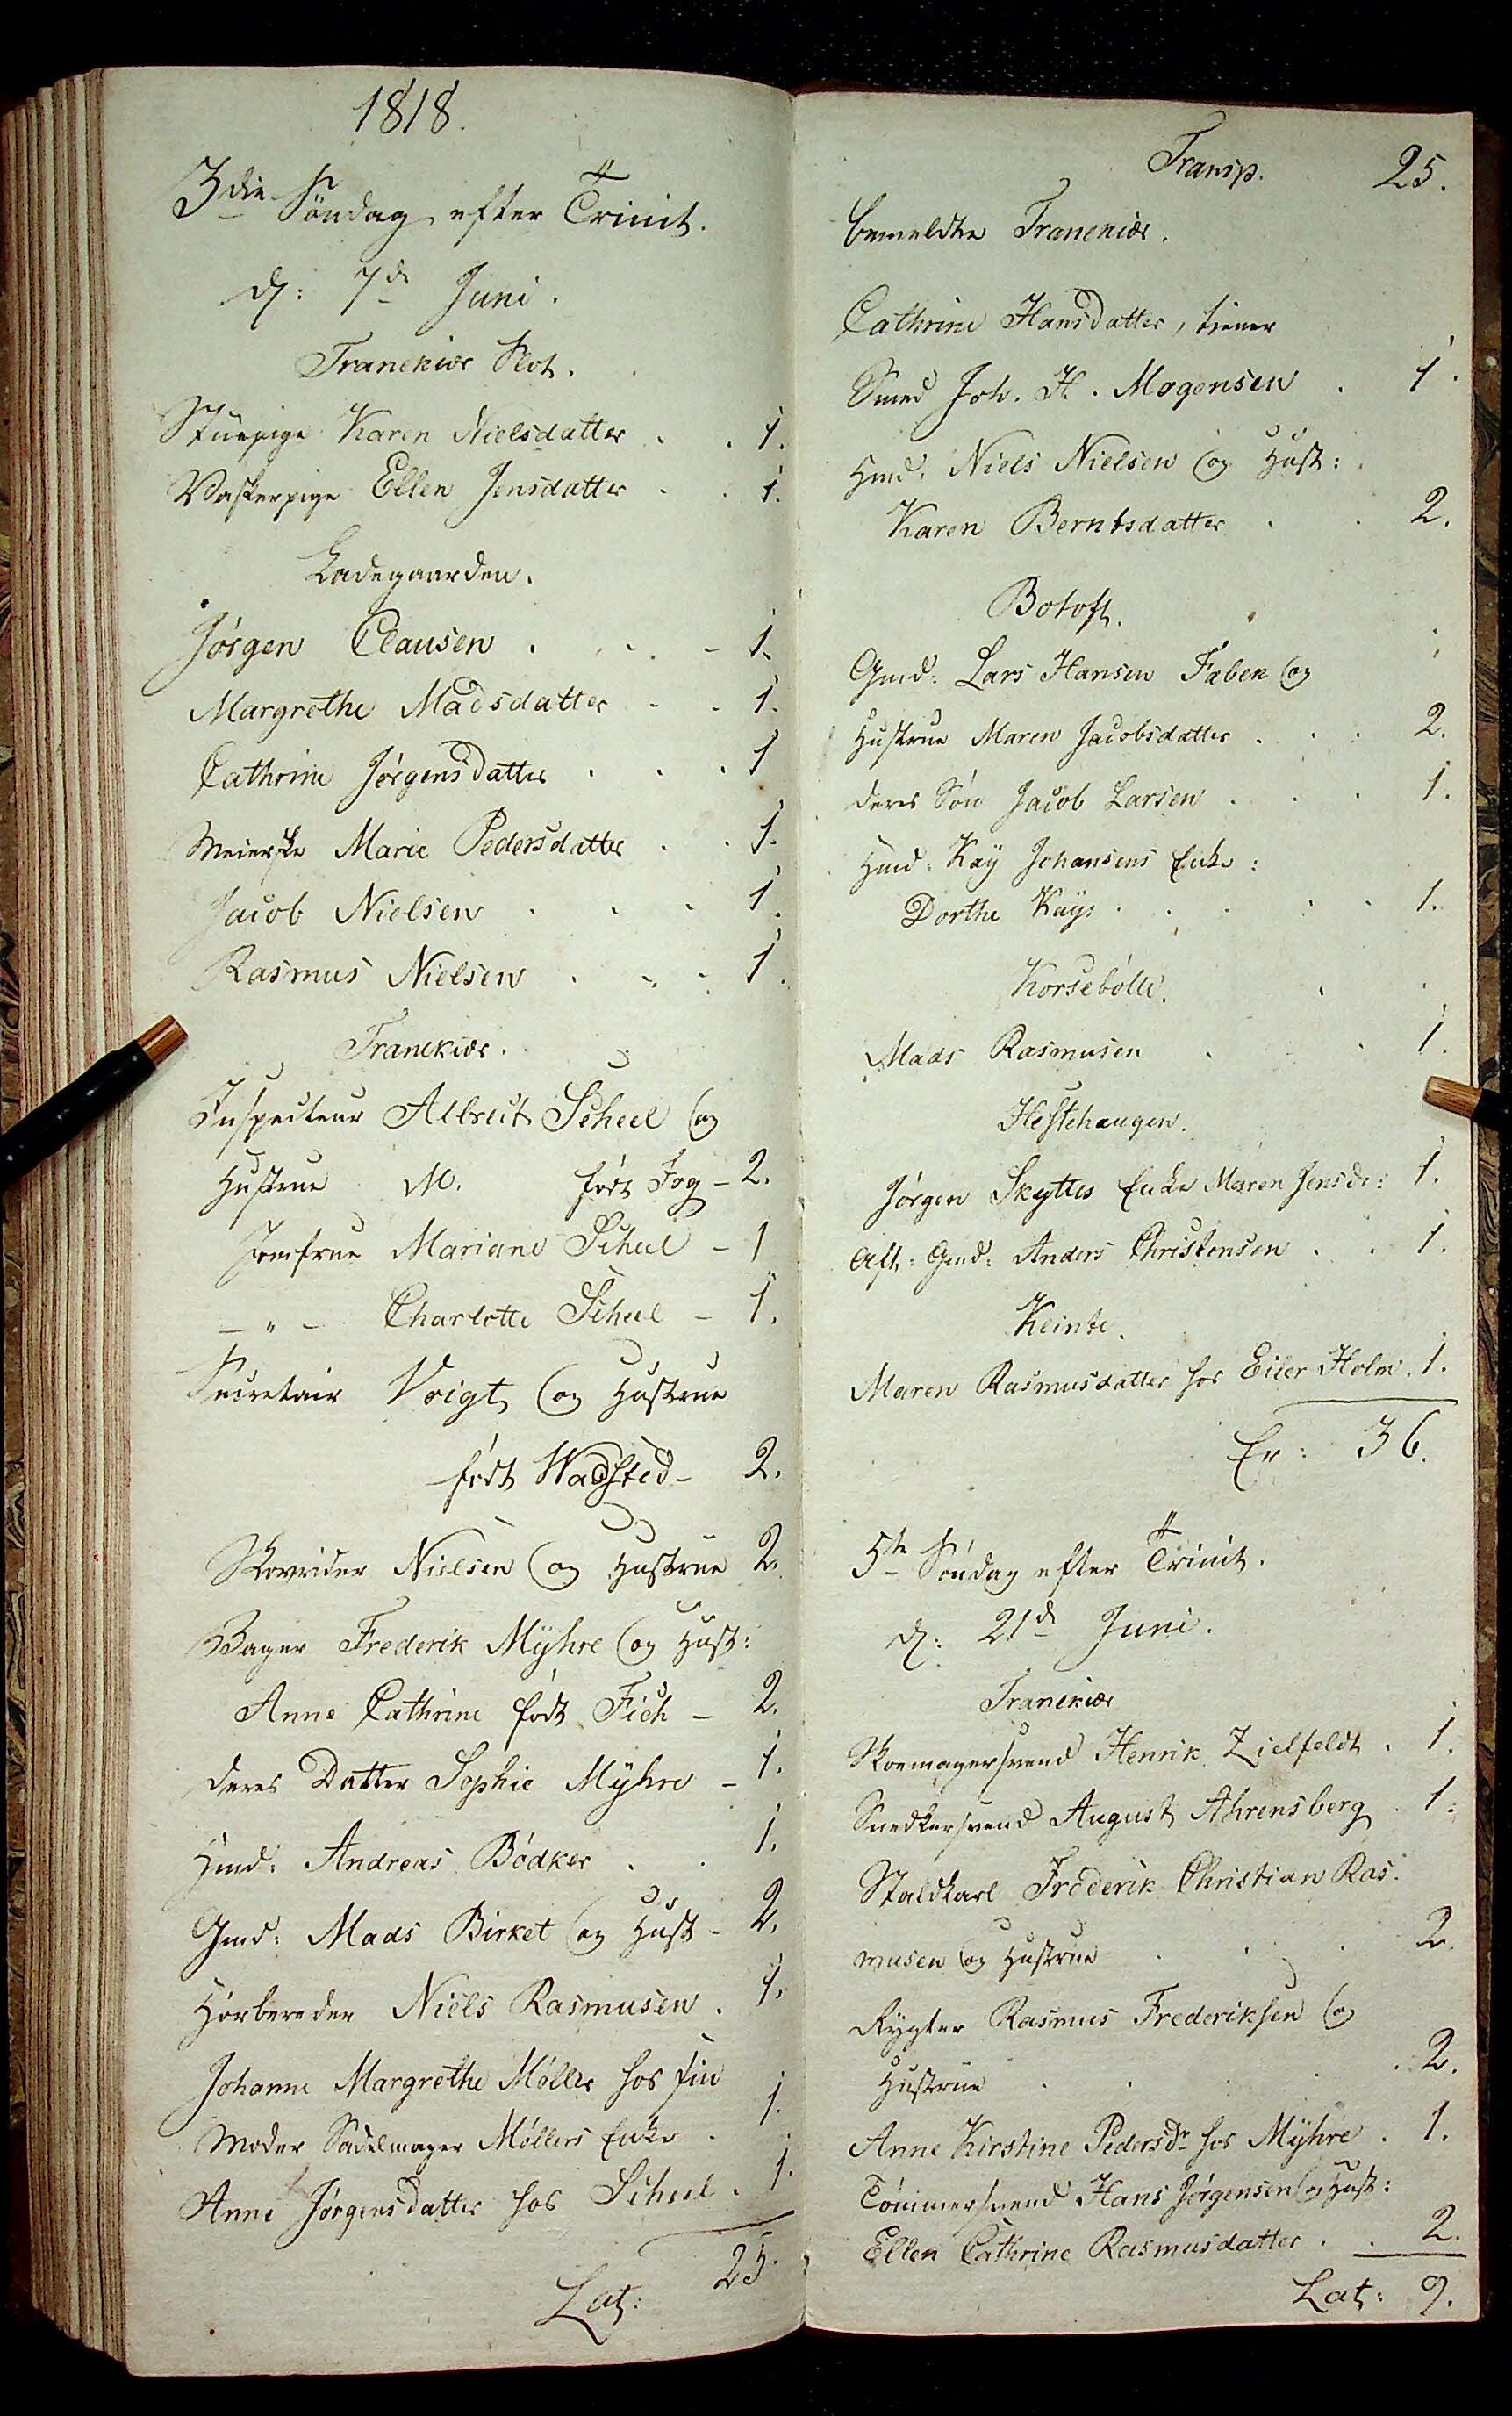1818.
3die Søndag efter Trinit.
d: 7de Juni.
Tranekiær Slot.
Stuepige Karen Nielsdatter . .1.
Vaskerpige Ellen Jensdatter . . 1.
Ladegaarden.
Jørgen Clausen . . . - 1.
Margrethe Madsdatter  . . 1.
Cathrine Jørgensdatter . . . 1.
Meierske Marie Pedersdatter . . 1.
Jacob Nielsen . . . 1.
Rasmus Nielsen . . . 1.
Tranekiær.
Inspecteur Albrect Scheel og
Hustrue M. født Fog - 2.
Jomfrue Mariane Scheel - 1
-"- Charlotte Scheel - 1.
Secretair Voigt og Hustrue
født Wadsted - 2.
Skovrider Nielsen og Hustrue 2.
Bager Frederik Myhre og Hust:
Anne Cathrine født Fich - 2.
deres Datter Sophie Myhre - 1.
Hmd: Andreas Bødker . . 1.
Gmd: Mads Birket og Hust - 2.
Hørbereder Niels Rasmusen . 1.
Johanne Margrethe Møller hos sin
Moder Sadelmager Møllers Enke . 1.
Anne Jørgensdatter hos Scheel . 1.
Lat: 25.
Transp. 25.
bemeldte Tranekiær.
Cathrine Hansdatter, tiener
Smed Joh. H. Mogensen . 1.
Hmd: Niels Nielsen og Hust:
Karen Berntsdatter . . 2.
Botoft.
Gmd: Lars Hansen Fæbek og
Hustrue Maren Jacobsdatter . . . 2.
deres Søn Jacob Larsen . . . 1.
Hmd: Kay Johansens Enke:
Dorthe Kays . . . . . 1.
Korsebølle.
Mads Rasmusen . . 1.
Hestehaugen.
Jørgen Skyttes Enke Maren Jensdr : 1.
Aft: Gmd: Anders Christensen . . 1.
Klinte.
Maren Rasmusdatter hos Eiler Holm . 1.
Er: 36.
5te Søndag efter Trinit.
d:
21de Juni.
Tranekiær
Skomagersvend Henric Zielfeldt . 1.
Snedkersvend August Ahrensberg . 1.
Staldkarl Friderik Christian Ras¬
musen og Hustrue . . . 2.
Rygter Rasmus Frederiksen og
Hustrue . . . . . 2.
Anne Kirstine Pedersdr hos Myhre . 1.
Tømmersvend Hans Jørgensen og Hust:
Ellen Cathrine Rasmusdatter . . 2.
Lat: 9.
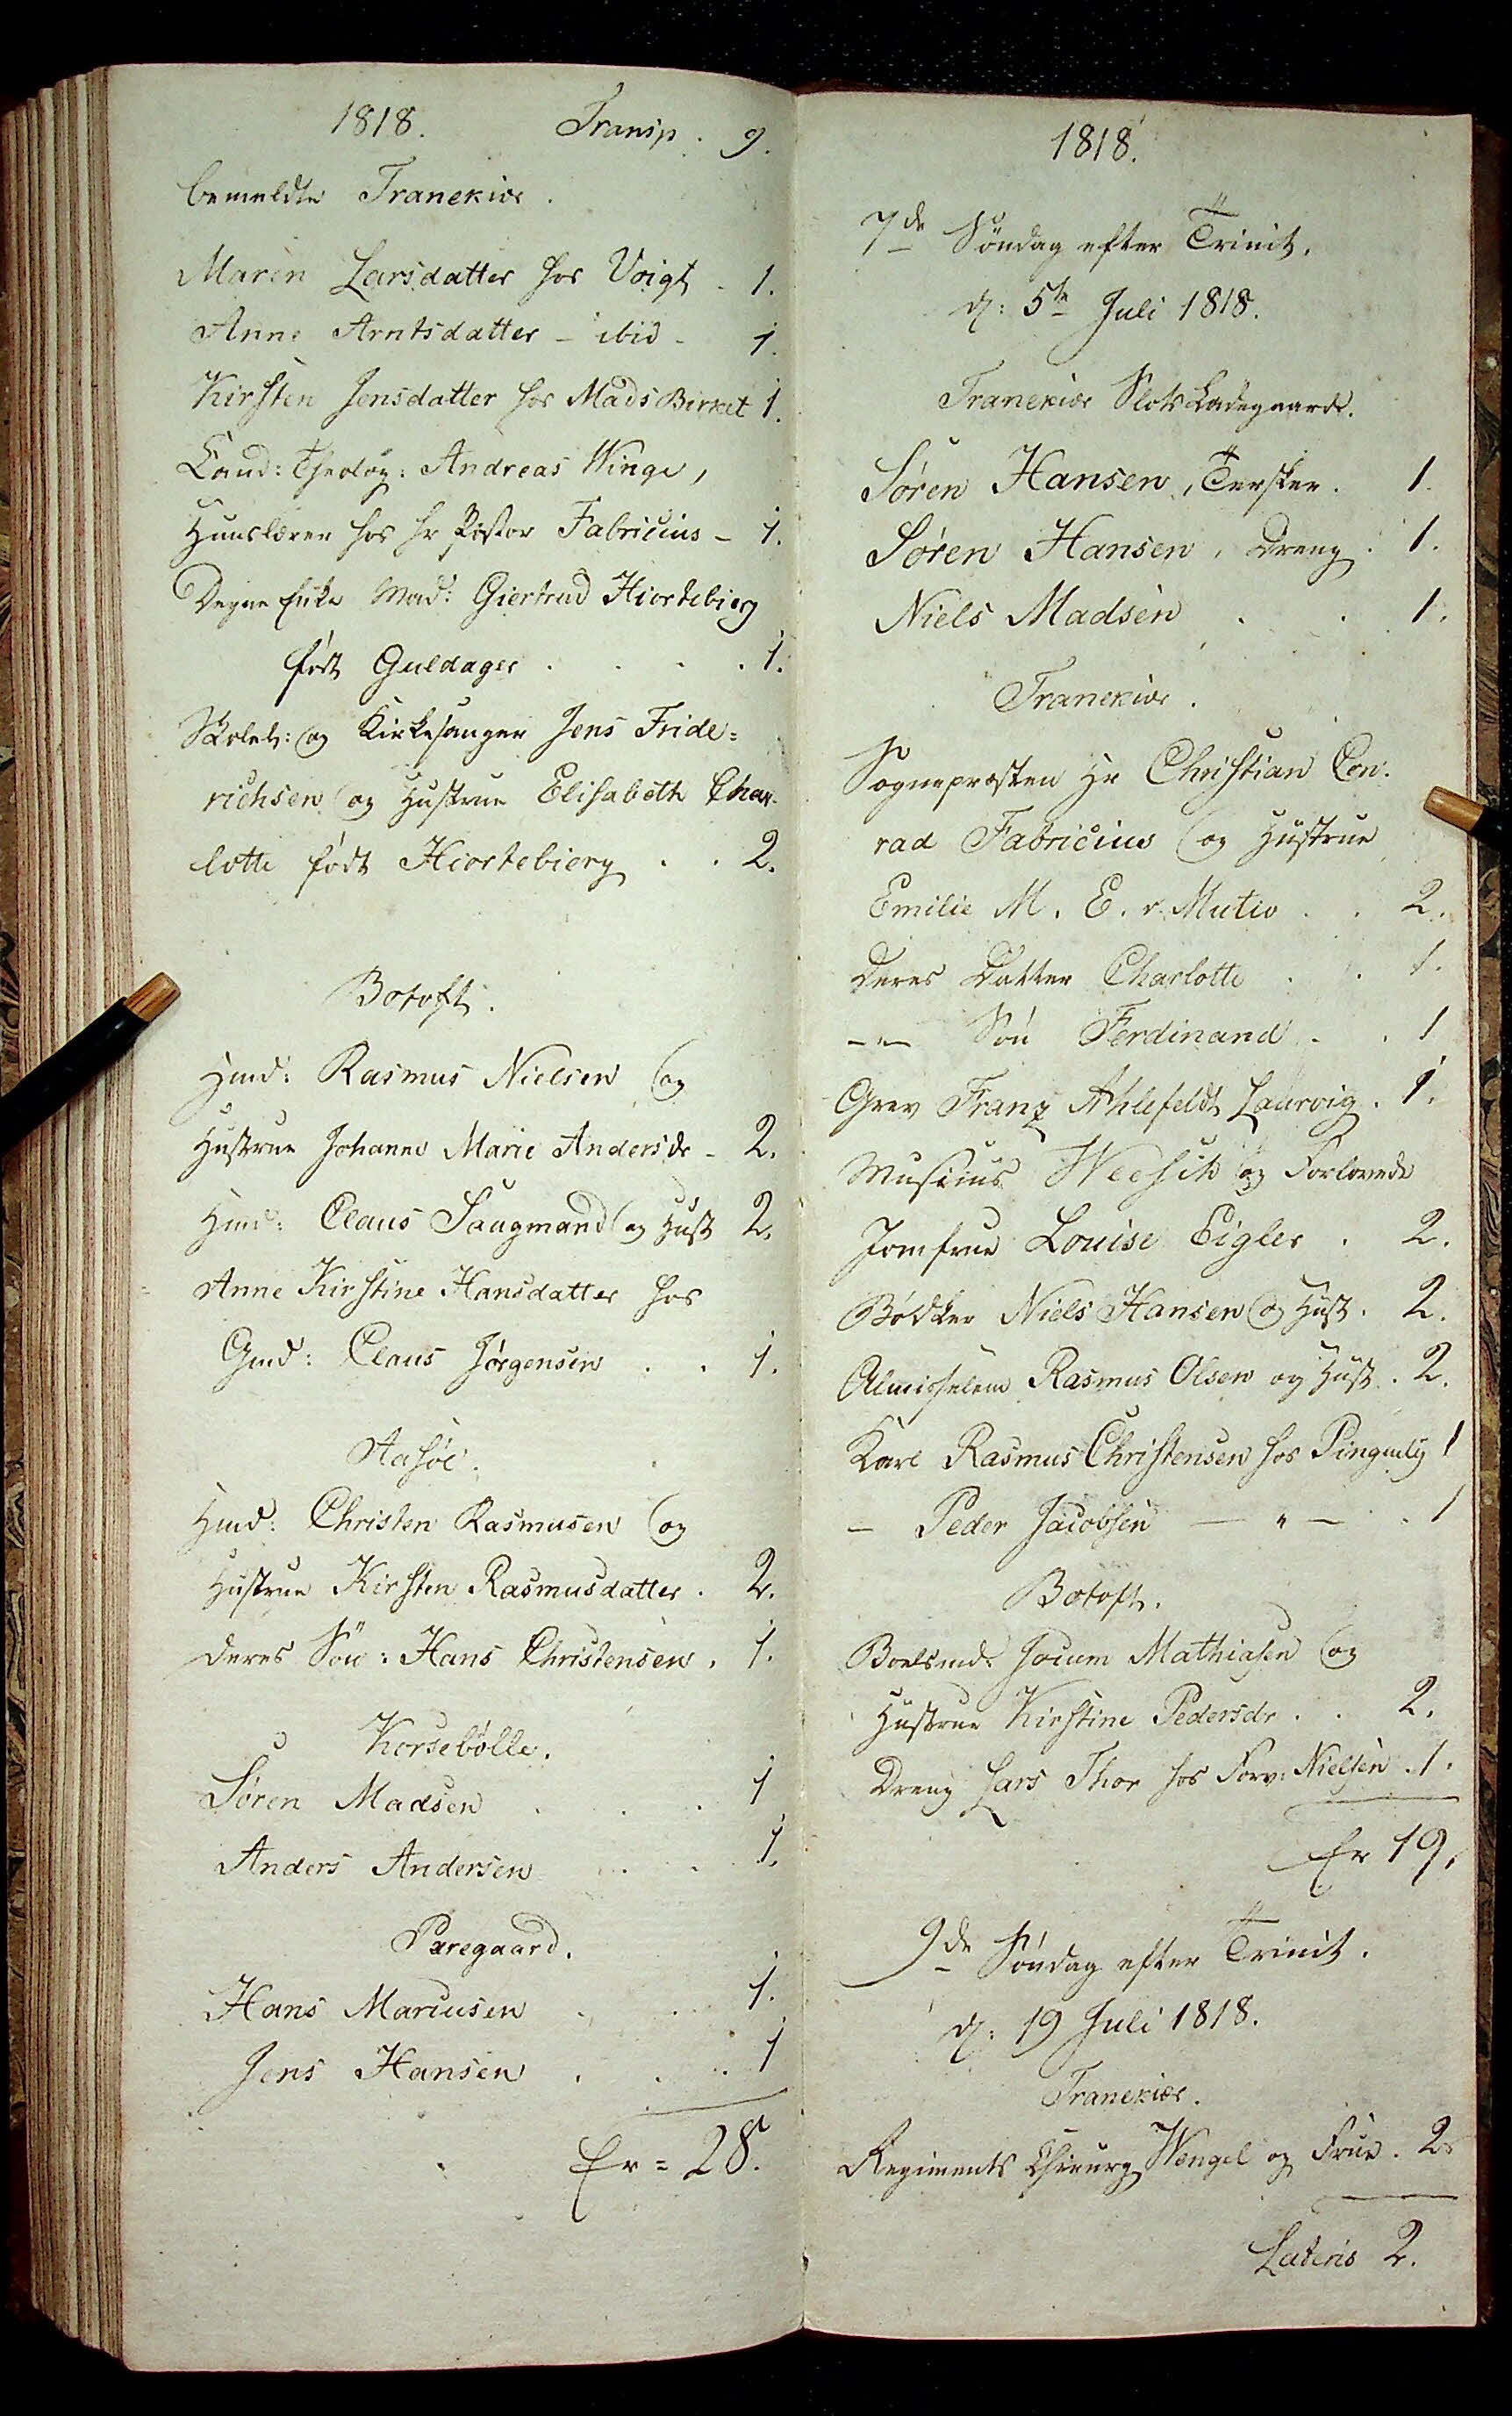1818.
Transp. 9.
bemeldte Tranekiær
Maren Larsdatter hos Voigt . 1.
Anne Arntsdatter - ibid - 1.
Kirsten Jensdatter hos Mads Birket 1.
Cand: Theolog: Andreas Winge,
Huuslærer hos sr Pastor Fabricius - 1.
Degne Enke Mad: Giertrud Hiortebierg
født Guldager . . . . 1.
Skolel: og Kirkesanger Jens Fride¬
richsen og Hustrue Elisabeth Char¬
lotte født Hiortebierg . . 2.
Botoft.
Hmd: Rasmus Nielsen og
Hustrue Johanne Marie Andersdr - 2.
Hmd: Claus Saugmand og Hust 2.
Anne Kirstine Hansdatter hos
Gmd: Claus Jørgensøn .  . 1.
Aasøe.
Hmd: Christen Rasmusen og
Hustrue Kirsten Rasmusdatter . 2.
deres Søn: Hans Christensen . 1.
Korsebølle.
Søren Madsen . . . 1
Anders Andersen . . . 1.
Pæregaard.
Hans Marcusen . . 1.
Jens Hansen . . . 1
Er = 28.
1818.
7de Søndag efter Trinit.
d: 5te Juli 1818.
Tranekiær Slots Ladegaard.
Søren Hansen Terskter . 1.
Søren Hansen, Dreng . 1.
Niels Madsen . . 1.
Tranekiær.
Sognepræsten Hr Christian Con¬
rad Fabricius og Hustrue
Emilie M. E. v. Mutio . . 2.
deres Datter Charlotte . . 1.
-"- Søn Ferdinand . . 1
Grev Franz Ahlefeldt Laurvig . 1.
Musicus Weesih og Forlovede
Jomfrue Louise Eigler . 2.
Bødker Niels Hansen og Hust . 2.
Almisselem Rasmus Olsen og Hust . 2.
Karl Rasmus Christensen hos Pinguely 1
- Peder Jacobsen -"- 1
Botoft.
Boelsmd. Jocum Mathiasen og
Hustrue Kirstine Pedersdr . .  2.
Dreng Lars Thor hos Forv: Nielsen . 1.
Er 19.
9de Søndag efter Trinit.
d: 19 Juli 1818.
Tranekiær.
Regiments Chirurg Wengel og Frue . 2.
Lateris 2.